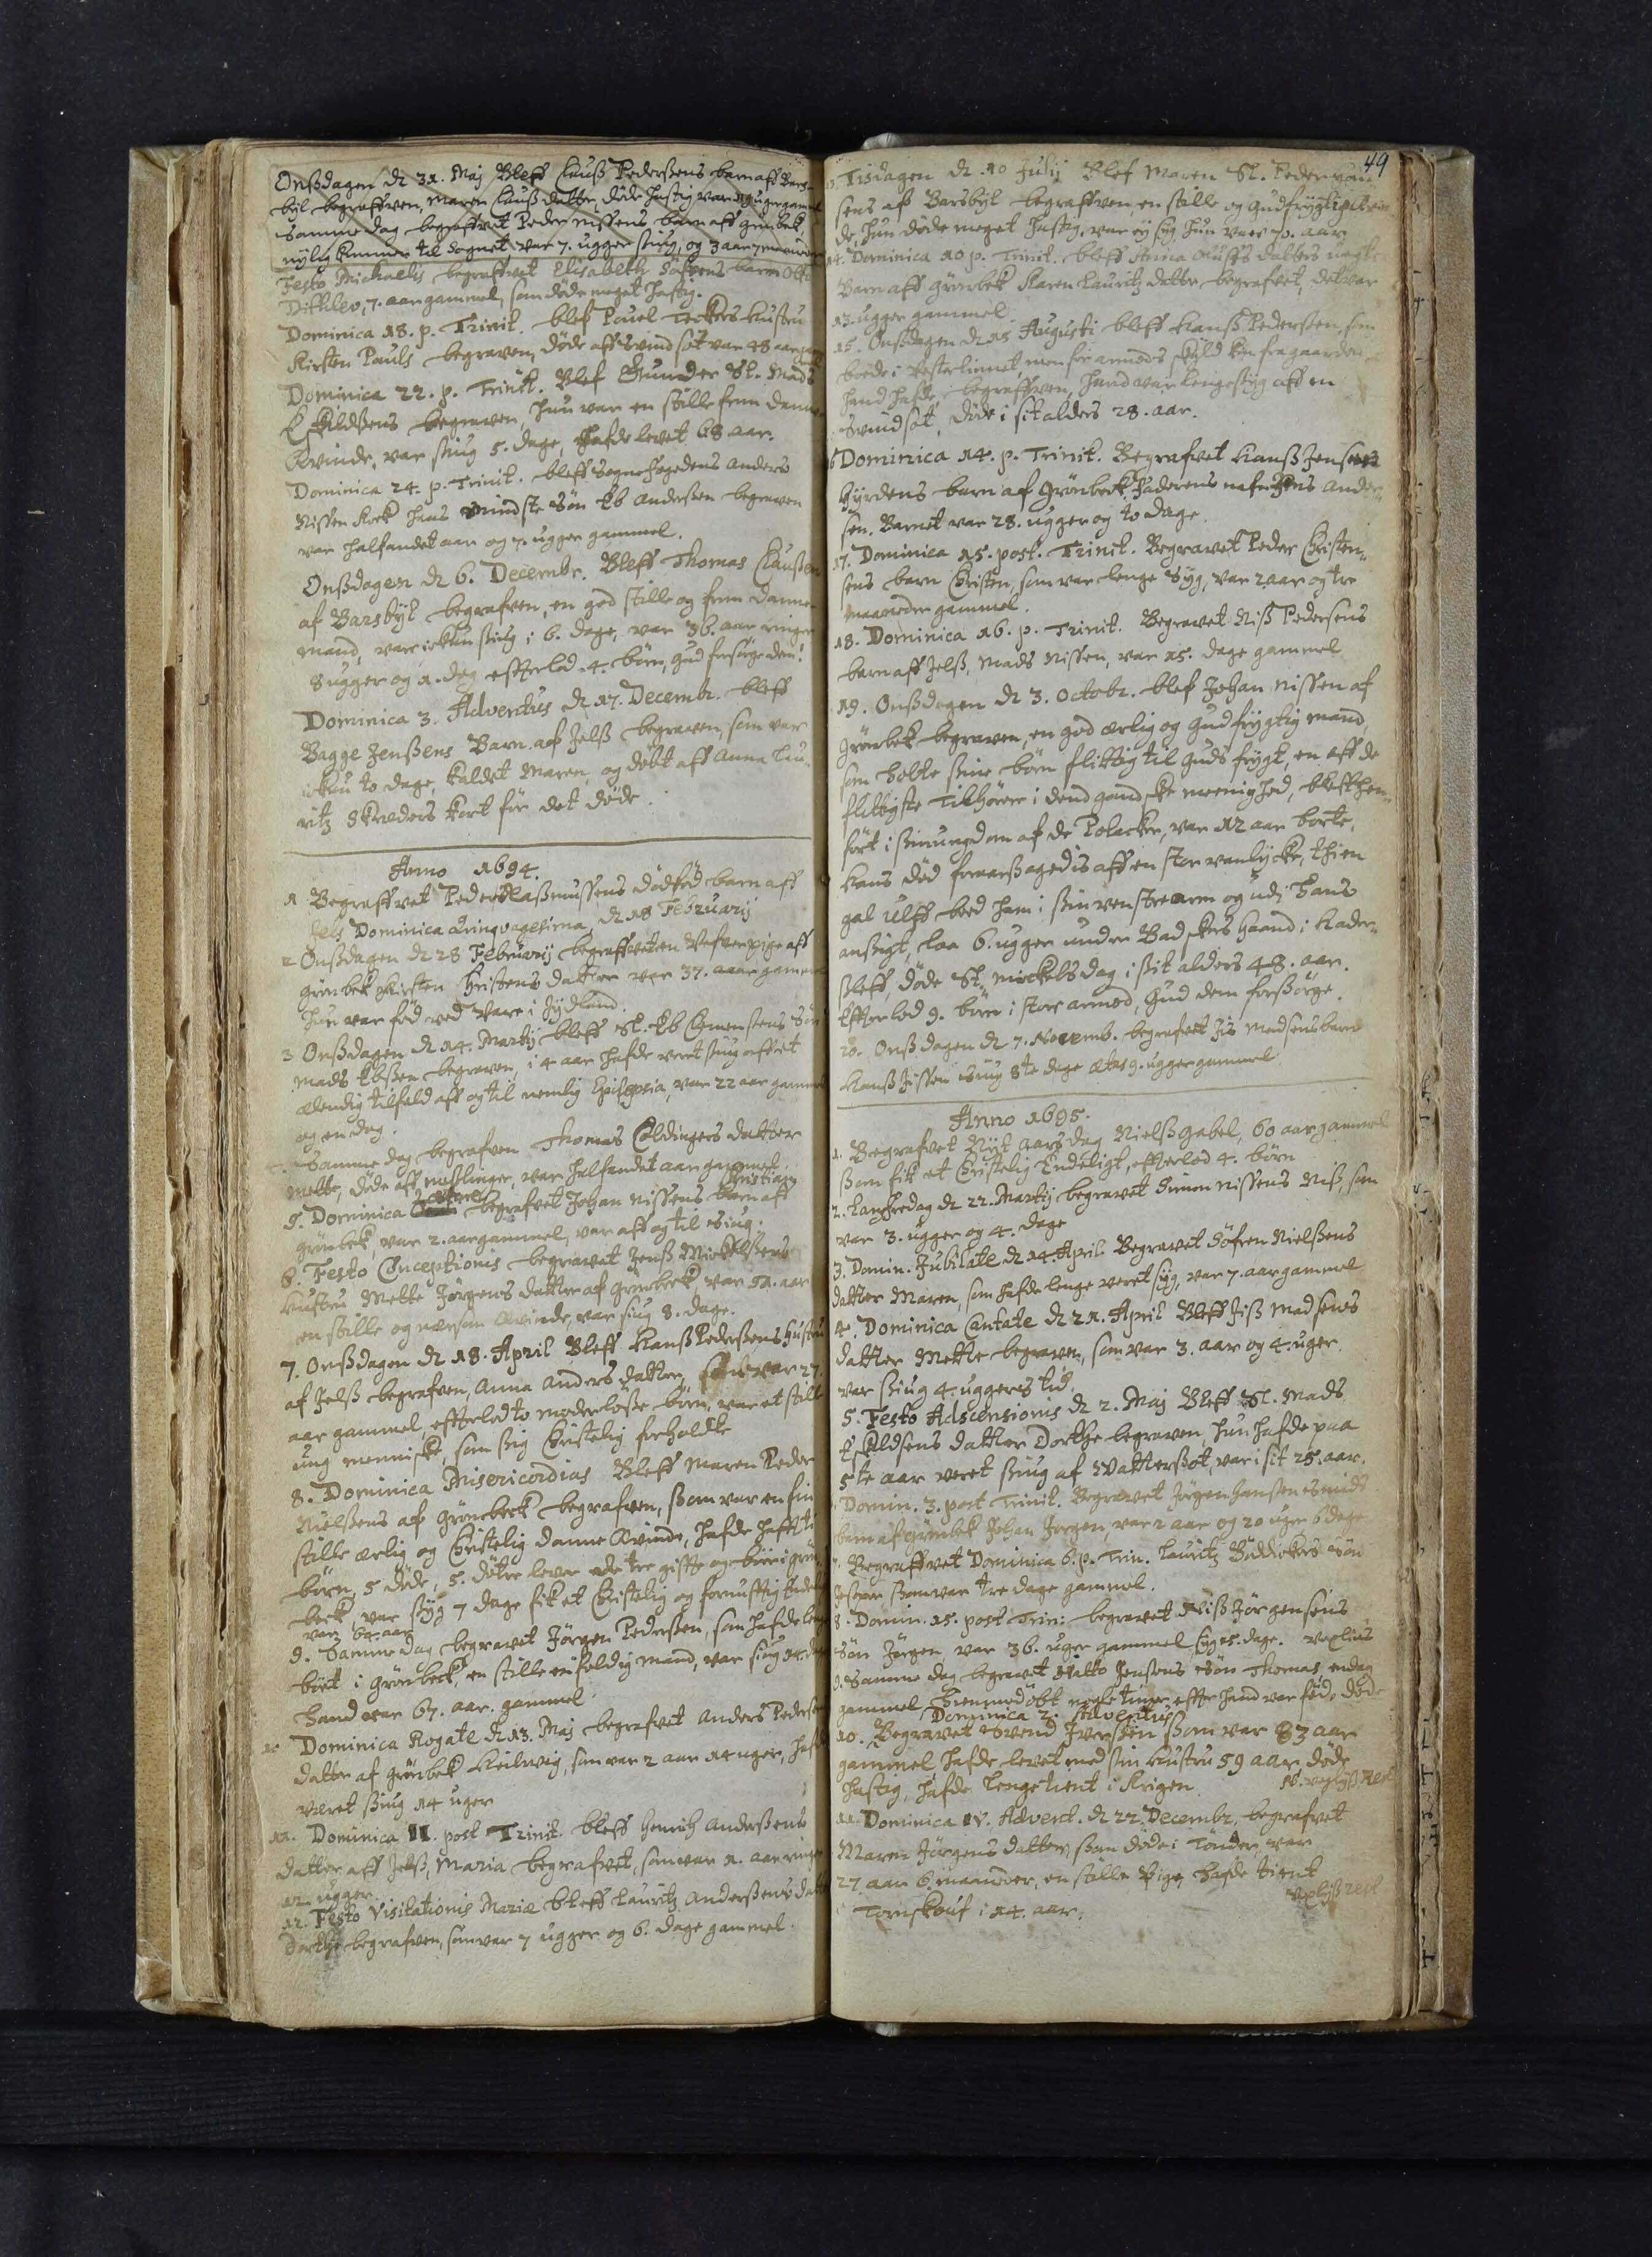Onssdagen d 31. maj Bleff Clauss Pederessens barn aff Bars¬
byl begraffven, Maren Clauss Datter, døde hastig var 19 ug gammel
Samme Dag begraffvet Peder Nissens barn aff Grønbek
mylig kommen til Sognet var 7, ugger suig, og 3 aar 7 maaneder
Festo Michaelis begraffvet Elisabeth Søfrens barn Otte
Dithlev, 7. aar gammel, som døde meget hastig.
Dominica 18. p. Trinit. blef Pouel Teckers hustru
Kirsten Pauls begraven, døde aff Svindsot var 48 aar g##
Dominica 22. p. Trinit. Blef Gunder Sl. Mads
Eskildssens begraven, hun var en stille from Danne
Qvinde, var siug 5. dage, hafde levet 68 aar.
Dominica 24. p. Trinit. bleff Sognefogedens Anders
Nissen Kock hans mindste Søn Eb Anderssen begraven
var halfandet aar og 7. ugger gammel.
Onsdagen d 6. Decembr. Bleff Thomas Clausen
af Barsbyl begrafven, en god stille og from Danne¬
mand, var ickun siug i 6. Dage, var 36. aar ringer
8 ugger og 1. dag efterlod 4. børn, Gud forsørge dem!
Dominica 3. Adventus d 17. Decembr. bleff
Bagge Jenssens Barn aff Jelss begraven, som var
ickun to dage, kaldet Maren, og døbt aff Anna Lau
ritz Skreders kort før det døde.
Anno 1694.
1. Begraffvet Peder Rassmussens dødfød barn aff
Jels Dominica Qvinqvagesima, d 18 Februarij
2. Onsdagen d 28 Februarij begraffvet en Vefverpige aff
Grønbek Kirsten Christens Datter var 37. aar gammel
hun var fød ved V## i Jydland.
3. Onssdagen d 14 Martij bleff Sl. Eb Clementsens Søn
Mads Ebssen begraven, i 4 aar hafde veret siug aff et
ælendig tilfald aff og til nemlig Episgoria##, var 22 aar gammel
og en Dag.
4. Samme dag begrafven Thomas Coldingers datter
Mette, døde aff meslinger, var halfandet aar gammel.
Christian
Lætare
5. Dominica O## begrafvet Johan Nissens barn aff
Grønbeck, var 2. aar gammel, var af og til Siug:
6. Festo Conceptionis begravet Jenss Mickelsens
Hustru Mette Jørgens datter af Grønbeck, var 51. aar
en stille og nænsom Qvinde, var siug 8. Dage.
7. Onssdagen d 18. April Bleff Hanss Pedersens Hustru
aff Jelss begrafven, Anna Anders datter, som var 27.
aar gammel, effterlod to moderløse børn, var et stille
ung menniske, som ssig Christelig forholdte
8. Dominica Misericordias Bleff Maren Peder
Nielssens aff Grønbeck begrafven, ssom var en fin
stille ærlig og Christelig Danne Qvinde, hafde haft ti
børn, 5 døde, 5. døtre leve ale tre giffte og boer i Grøn
beck, var ssyg 7 Dage fik et Christelig og fornufftig endeli
var 64 aar
9. Samme Dag begravet Jørgen Pederssen, som hafde leng
boet i Grønbeck, en stille enfoldig mand, var siug 14. dage
hand var 67. aar gammel
10. Dominica Rogate d 13. Maj begrafvet Anders Pedersens
datter aff Grønbeck Heilwig, som var 2 aar 14 uger, hafde
veret ssiug 14 uger
11. Dominica II. post Trinit. bleff Henrich Anderssens
datter aff Jelss, Maria, begrafvet, som var 1. aar ringer
12. ugger.
12. Festo Visitationis Mariæ bleff Lauritz Anderssens datt 
Dorthe begrafven, som var 7 ugger og 6. dage gammel.
49
13. Tisdagen d 10 Julij Bleff Maren Sl. Peder Han
sens aff Barsbyl begrafven en stille og gudfrygtig Qvin
de, hun døde meget hastig, var ey siug hun var 70. aar.
14. Dominica 10 p. Trinit. bleff Anna Oluffs datters uegte
Barn aff Grønbek Karen Lauritz datter begrafvet, det var
13. ugger gammel.
15. Onssdagen d 15 Augusti bleff Hanss Pederssen, som
boede i Vesterlinnet, men for armods skyld kom## fra gaarden
han hafde, begraffven, hand var lenge siug aff en
Svindsot, døde i sit alders 28. aar.
16. Dominica 14. p. Trinit. Begrafvet Hanss Jensens
Hyrdens barn aff Grønbeck. Faderens nafn Jens Ander¬
sen. Barnet var 28. ugger og to dage.
17. Dominica 15. post Trinit. Begravet Peder Christen¬
sens barn Christen, som var lenge Syg, var 2 aar og tre
maaneder gammel.
18. Dominica 16. p. Trinit. Begravet Niss Pedersens
barn aff Jelss, Mads Nissen, var 25. dage gammel
19. Onssdagen d 3. Octobr. blef Johan Nissen af
Grønbeck begraven, en god ærlig og gudfrygtig mand
som holte sine børn flittig til guds frygt, en aff de
flitigste Tilhører i dend gandske meenighed, bleff hen¬
ført i sin ungdom af de Polacker, var 12 aar borte,
hans død foraarssagedis aff en stor vanlycke, thi en
gal ulff beed ham i ssin venstre arm og udi hands
ansigt, laa 6. ugger under Badskers haand i Hader¬
sleff, døde St Mickelsdag i ssit alders 48. aar.
Efterlod 9. børn i stor armod, Gud dem forssørge.
20. Onsdagen d 7. Novemb. begrafet Jes Madsens barn
Hans Jessen siug 8te dage ætat## 9. ugger gammel.
Anno 1695
1. Begrafvet Nyt aars dag Nielss Gabel, 60 aar gammel
som fick et Christelig Endeligt, efterlod 4. børn
2. Langfredag d 22. Martij begravet Simon Nissens Niss, som
var 3. ugger og 4. dage
3. Domin. Jubilate d 14. April Begravet Søfren Nielssens
datter Maren, som hafde lenge veret syg, var 7. aar gammel.
4. Dominica Cantate d 21. April Bleff Jiss Madsens
datter Mette begraven, som var 3. aar og 4. uger.
Var ssiug 4. uggers tid.
5. Festo Adscensionis d 2. Maj Bleff Sl. Mads
Eskildsens datter Dorthe begraven, hun hafde paa
5te aar veret ssiug af Vattersot, var i sit 25. aar.
6. Domin. 3. post Trinit. Begravet Jurgen Hansen Smids
barn aff Grønbek Johan Jorgen, var 2 aar og 20 uger 6 dage.
7. Begraffvet Dominica 6. p. Trin. Lauritz Bøddickers Søn
Jesper som var tre Dage gammel.
8. Domin. 15. post Trin: begravet Niss Jørgensens
Søn Jørgen var 36. uger gammel siug 15 dage. 
9. Samme Dag begravet Hatto Jensens Søn Thomas en dag
gammel, hiemedøbt nogle timer efter hand var fød, døde
Dominica 2. Adventus
10. Begravet Svend Iverssen ssom var 83 aar
gammel hafde levet med sin Hustru 59 aar døde
hastig, hafde lenge tient i Krigen.
11. Dominica IV. Advent. D 22 Decembr. begrafvet
Maren Jørgens Datter, ssom døde i Tønder, var
27 aar 6. maaneder, en stille Pige, hafde tient
Torskouf i 14 aar.